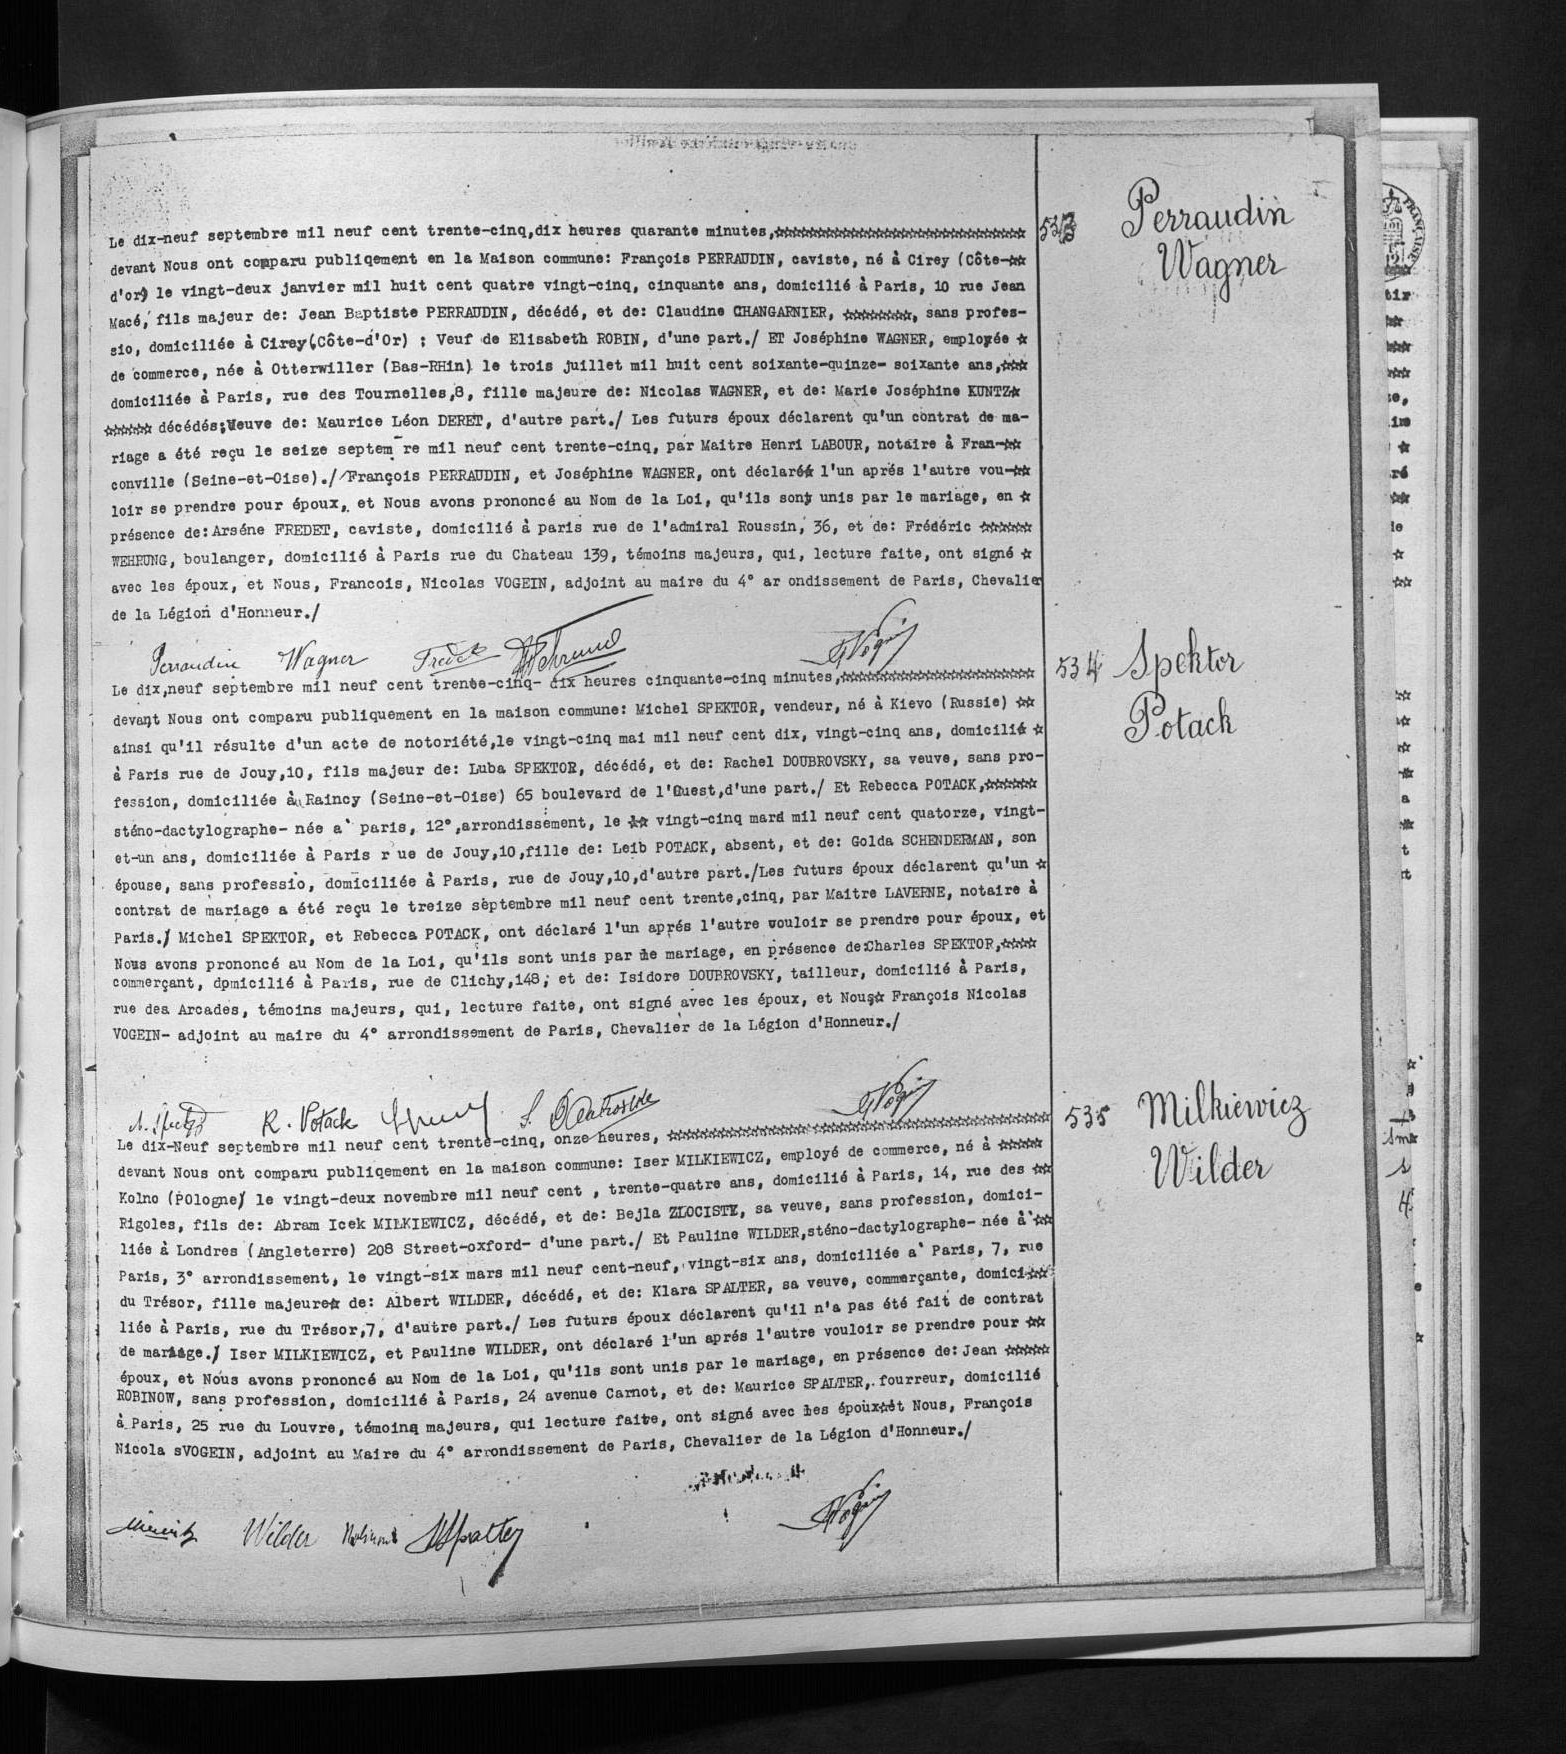Le dix-neuf septembre mil neuf cent trente-cinq, dix heures quarante minutes, ****
devant Nous ont comparu publiqement en la Maison commune: François PERRAUDIN, caviste, né à Cirey (Côte -**
d'or) le vingt-deux janvier mil huit cent quatre vingt-cinq, cinquante ans, domicilié à Paris, 10 rue Jean
Macé, fils majeurs de: Jean Baptiste PERRAUDIN, décédé, et de: Claudine CHANGARNIER, ****, sans profes-
sio, domicilié à Cirey (Côte-d'Or); Veuf de Elisabeth ROBIN, d'une part ./ ET Joséphine WAGNER, employée *
de commerce, née à Otterwiller (Bas-RHin) le trois juillet mil huit cent soixante-quinze soixante ans ,***
domiciliée à Paris, rue des Tournelles, 8, fille majeure de: Nicolas WAGNER, et de: Marie Joséphine KUNTZ*
**** décédés; Veuve de: Maurice Léon DERET, d'autre part ./ Les futurs époux déclarent qu'un contrat de ma-
riage a été reçu le seize septem re mil neuf cent trente-cinq, par Maitre Henri LABOUR, notaire à Fran -**
conville (Seine-et-Oise) ./ François PERRAUDIN, et Joséphine WAGNER, ont déclaré * l'un aprés l'autre vou -**
loir se prendre pour époux, et Nous avons prononcé au Nom de la Loi, qu'ils sont unis par le mariage, en *
présence de: Arséne FREDET, caviste, domicilié à paris rue de l'admiral Roussin, 36, et de: Fréderic ****
WEHRUNG, boulanger, domicilié à Paris rue du Chateau 139, témoins majeurs, qui, lecture faite, ont signé *
avec les époux, et Nous, François, Nicolas VOGEIN, adjoint au maire du 4° ar ondissement de Paris, Chevalier
de la Légion d'Honneur ./
Le dix, neuf septembre mil neuf cent trence-cinq-dix heures cinquante-cinq minutes, ****
devant Nous ont comparu publiquement en la maison commune: Michel SPEKTOR, veudeur, né à Kievo (Russie) **
ainsi qu'il résulte d'un acte de notoriété, le vingt-cinq mai mil neuf cent dix, vingt-cinq ans, domicilié *
à Paris rue de Jouy, 10, fils majeur de: Luba SPEKTOR, décédé, et de: Rachel DOUBROVSKY, sa veuve, sans pro-
fession, domiciliée à Raincy (Seine-et-Oise) 65 boulevard de l'Ouest, d'une part ./ Et Rebecca POTACK ,****
sténo-dactylographe-née à Paris r ue de Jouy, 10, fille de: Leib POTACK, absent, et de: Golda SCHENDERMAN, son
épouse, sans professio, domiciliée à Paris, rue de Jouy, 10, d'autre part ./ Les futurs époux déclarent qu'un *
contrat de mariage a été reçu le treize septembre mil neuf cent trente, cinq, par Maitre LAVERNE, notaire à
Paris ./ Michel SPEKTOR, et Rebecca POTACK, ont déclaré l'un aprés l'autre vouloir se prendre pour époux, et
Nous avons prononcé au Nom de la Loi, qu'ils sont unis par me mariage, en présence de Charles SPEKTOR ,****
commerçant, domicilié à Paris, rue de Clichy, 148; et de: Isidore DOUBROVSKY, tailleur, domicilié à Paris,
rue des Arcades, témoins majeurs, qui, lecture faite, ont signé avec les époux, et Nous * François Nicolas
VOGEIN-adjoint au maire du 4° arrondissement de Paris, Chevalier de la Légion d'Honneur ./
Le dix-Neuf septembre mil neuf cent trente-cinq, onze heures, ****
devant Nous ont comparu publiqement en la maison commune: Iser MILKIEWICZ, employé de commerce, né à ****
Kolno (Pologne) le vingt-deux novembre mil neuf cent, trente-quatre ans, domicilié à Paris, 14, rue des **
Rigoles, fils de: Abram Icek MILKIEWICK, décédé, et de: Bejla ZLOCISTY, sa veuve, sans profession, domici-
liée à Londres (Angleterre) 208 Street-Oxford-d'une part ./ Et Pauline WILDER, sténo-dactylogrpahe-née à **
Paris, 3° arrondissement, le vingt-six mars mil neuf cent-neuf, vingt-six ans, domiciliée à Paris, 7, rue
du Trésor, fille majeure * de: Albert WILDER, décédé, et de: Klara SPALTER, sa veuve, commerçante, domici **
liée à Paris, rue du Trésor, 7, d'autre part ./ Les futurs époux déclarent qu'il n'a pas été fait de contrat
de mariage ./ Iser MILKIEWICZ, et Pauline WILDER, ont déclaré l'un aprés l'autre vouloir se prendre pour **
époux, et Nous avons prononcé au Nom de la Loi, qu'ils sont unis par le mariage, en présence de: Jean ***
ROBINOW, sans profession, domicilié à Paris, 24 avenue Carnot, et de: Maurice SPALTER, fourreur, domicilié
à Paris, 25 rue du Louvre, témoinq majeurs, qui lecture faite, ont signé avec les époux * et Nous, François
Nicola sVOGEIN, adjoint au Maire du 4° arrondissement de Paris, Chevalier de la Légion d'Honneur ./
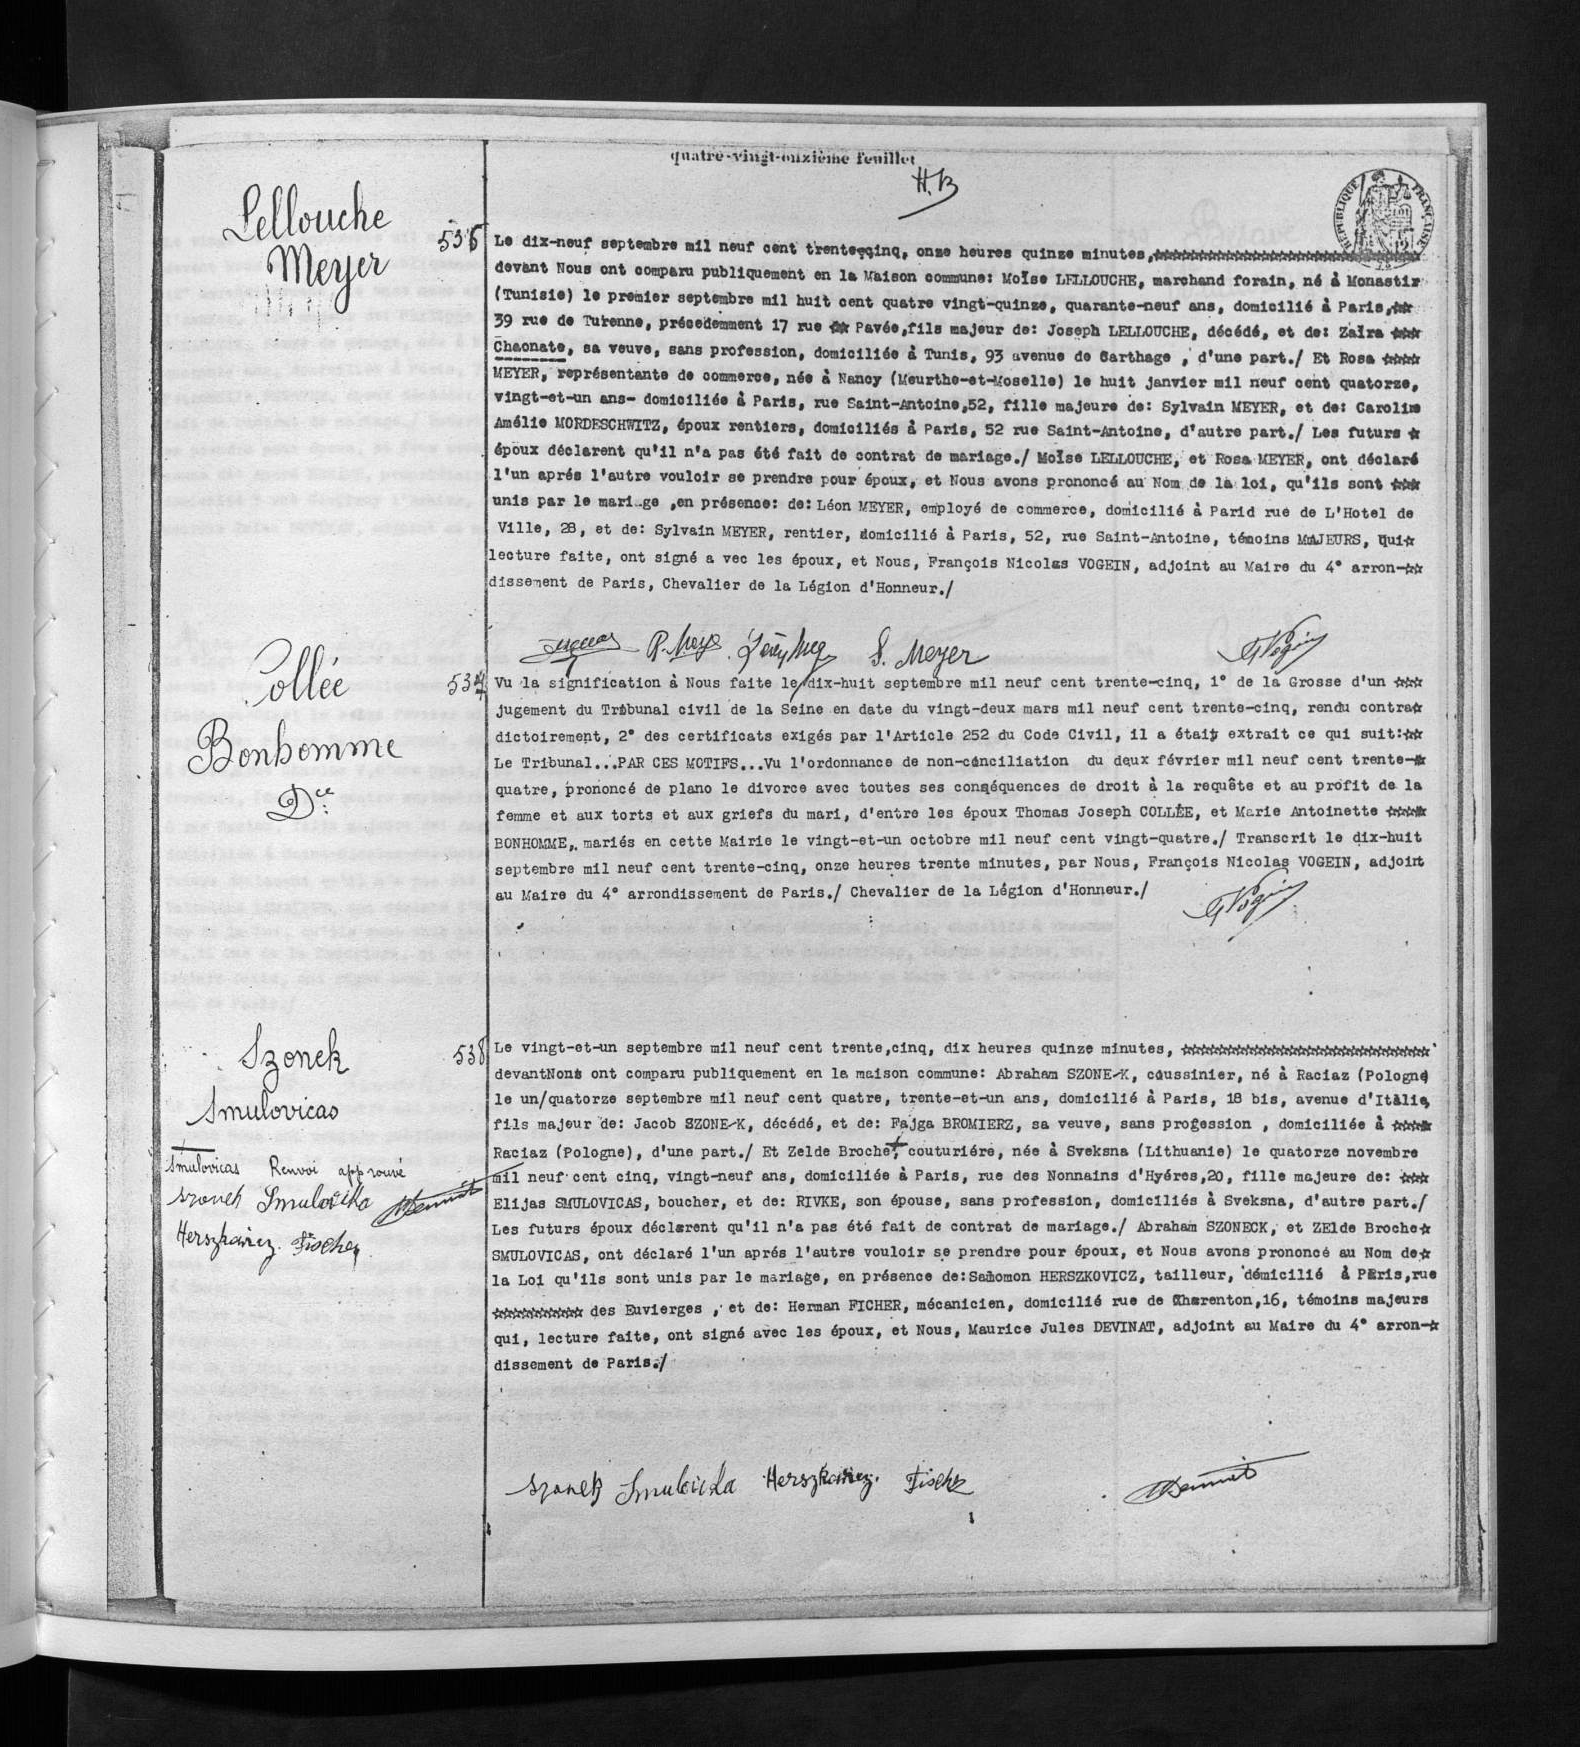Le dix-neuf septembre mil neuf cent trente cinq, onze heures quinze minutes, ****
devant Nous ont comparu publiquement en la Maison commune: Moïse LEFLOUCHE, marchand forain, né à Monsstir
(Tunisie) le premier septembre mil huit cent quatre vingt-quinze, quarante-neuf ans, domicilié à Paris ,**
39 rue de Turenne, précedemment 17 rue * Pavée, fils majeur de: Joseph LELLOUCHE, décédé, et de: Zaïra ***
Chaonate, sa veuve, sans profession, domiciliée à Tunis, 93 avenue de Carthage, d'une part ./ Et Rosa ***
MEYER, représentante de commerce, née à Nancy (Meurthe-et-Moselle) le huit janvier mil neuf cent quatorze,
vingt-et-un ans-domiciliée à Paris, rue Saint-Antoine, 52, fille majeure de: Sylvain MEYER, et de: Caroline
Amélie MORDESCHWITZ, époux rentiers, domiciliés à Paris, 52 rue Saint-Antoine, d'autre part ./ Les futurs *
époux déclarent qu'il n'a pas été fait de contrat de mariage ./ Moïse LELLOUCHE, et Rosa MEYER, ont déclaré
l'un aprés l'autre vouloir se prendre pour époux, et Nous avons prononcé au Nom de la loi, qu'ils sont ***
unis par le mariage, en présence: de: Léon MEYER, employé de commerce, domicilié à Parid rue de L'Hotel de
Ville, 28, et de: Sylvain MEYER, rentier, domicilié à Paris, 52, rue Saint-Antoine, témoins MAJEURS, qui *
lecture faite, ont signé a vec les époux, et Nous, François Nicolas VIGEIN, adjoint au Maire du 4° arron -**
dissement de Paris, Chevalier de la Légion d'Honneur ./
Vu la signification à Nous faite le dix-huit septembre mil neuf cent trente-cinq, 1° de la Grosse d'un ***
jugement du Tribunal civil de la Seine en date du vingt-deux mars mil neuf cent trente-cinq, rendu contra ***
dictoirement, 2° des certificats exigés par l'Article 252 du Code Civil, il a était extrait ce qui suit: **
Le Tribunal... PAR CES MOTIFS... Vu l'ordonnance de non-conciliation du deux février mil neuf cent trente-*
quatre, prononcé de plano le divorce avec toutes ses conséquences de-droit à la requête et au profit de la
femme et aux torts et aux griefs du mari, d'entre les époux Thomas Joseph COLLEE, et Marie Antoinette ****
BONHOMME, mariés en cette Mairie le vingt-et-un octobre mil neuf cent vingt-quatre ./ Transcrit le dix-huit
septembre mil neuf cent trente-cinq, onze heures trente minutes, par Nous, François Nicolas VOGEIN, adjoint
au Maire du 4° arrondissement de Paris ./ Chevalier de la Légion d'Honneur ./
Le vingt-et-un septembre mil neuf cent trente, cinq, dix heures quinze minutes, ****
devantNons ont comparu publiquement en la maison commune: Abraham SZONE K, coussinier, né à Raciaz (Pologne)
le un/ quatorze septembre mil neuf cent quatre, trente-et-un ans, domicilié à Paris, 18 bis, avenue d'Italie,
fils majeur de: Jacob SZONE K, décédé, et de: Fadja BROMIERZ, sa veuve, sans profession, domiciliée à ****
Raciaz (Pologne), d'une part ./ Et Zelde Borhce, couturiére, née à Svekana (Lithuanie) le quatorze novembre
mil neuf cent cinq, vingt-neuf ans, domiciliée à Paris, rue des Nonnains d'Hyéres, 20, fille majeure de: ***
Elijas SMULOVICAS, boucher, et de: RIVKE, son épouse, sans profession, domiciliés à Svekana, d'autre part ./
Les futurs époux déclarent qu'il n'a pas été fait de contrat de mariage ./ Abraham SZONECK, et ZEIde Broche *
SMULOVICAS, ont déclaré l'un aprés l'autre vouloir se prendre pour époux, et Nous avons prononcé au Nom de *
la Loi qu'ils sont unis par le mariage, en présence de: Salomon HERSZOVICK, tailleur, démicilié à Paris, rue
**** des Euvierges, et de: Herman FICHER, mécanicien, domicilié rue de Charenton, 16, témoins majeurs
qui, lecture faite, ont signé avec les époux, et Nous, Maurice Jules DEVINAT, adjoint au Maire du 4° arron -*
dissement de Paris ./